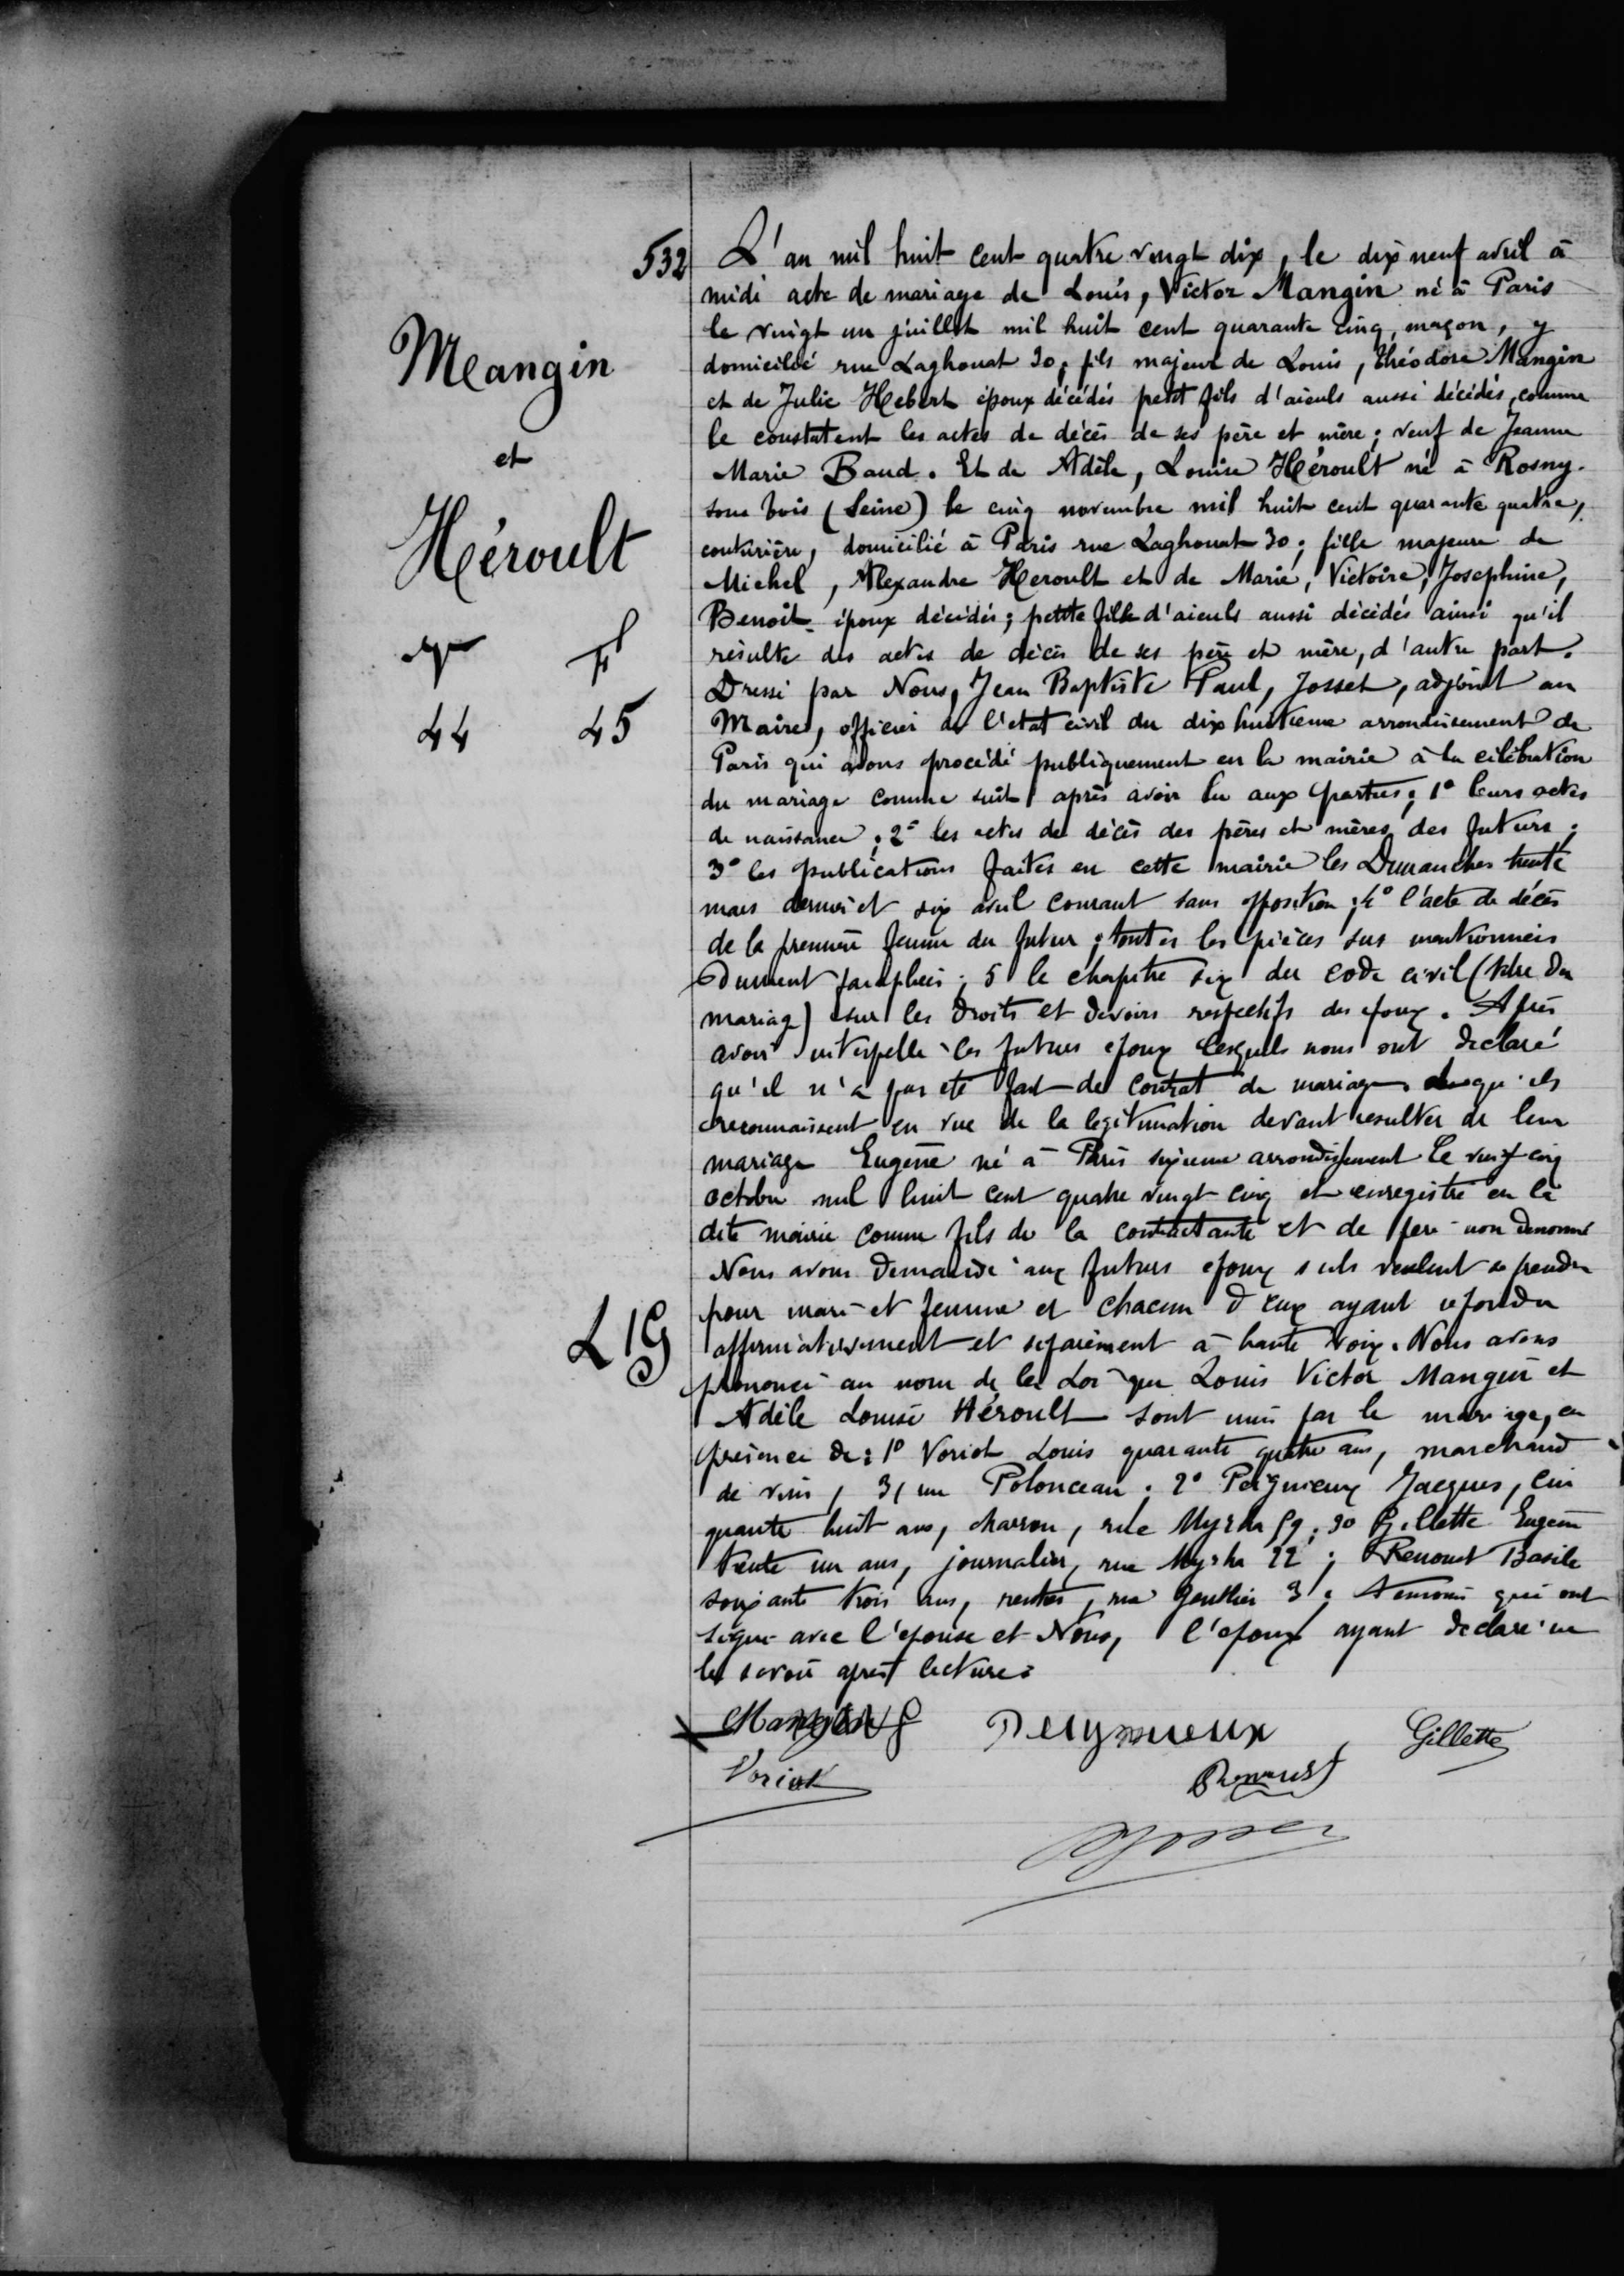532
Meangin
et
Héroult
V F
44 45
L'an mil huit cent quatre vingt dix, le dix neuf avril à
midi acte de mariage de Louis, Victor Mangin né à Paris
le vingt un juillet mil huit cent quarante cinq, maçon, y
domicilié rue Laghoust 90, fils majeur de Louis, Théodore Manfin
et de Julie Hebert époux décédés petit fils d'aieuls aussi décédé comme
le constatent les actes de décès de ses père et mère; veuf de Jeanne
Marie Baud. Et de Adèle, Louise Héroult né à Rosny
sous bois (Seine) le cinq novembre mil huit cent quarante quatre,
couturière, domicilié à Paris rue Laghoust 30. fille majeure de
Michel, Alexandre Heroult et de Marie, Victoire, Josephine,
Benoit époux déédés; petite fille d'aieuls aussi décédés ainsi qu'il
résulte des actes de décès de ses père et mère, d'autre part.
Dressé par Nous, Jean Baptiste Paul, Joseet, adjoint au
Maire, officier de l'etat civil du dix huitième arrondissement de
Paris qui avons procédé publiquement en la mairie à la célébration
du mariage comme suit après vaoir lu aux parties; 1° leurs actes
de naissances, 2° les actes de décès des pères et mères des futurs;
3° les publications faites en cette mairie les Dimanches trente
mars dernier et six avril courant sans opposition; 4° l'acte de décès
de la première femme du futur; toutes les pièces sus mentionnées
dument paraphées; 5 le chapitre six du code civil (titre du
mariage) sur les droits et devoirs respectifs des époux. Après
avoir interpellé les futurs époux lesquels nous ont déclaré
qu'il n'a pas été fait de contrat de mariage puisqu'ils
reconnaissant en vue de la légitimation devant resulter de leur
mariage Eugène né à Paris sixième arrondissement le vingt-cinq
octobre mil huit cent quatre vingt cinq et enregistré en la
dite mairie comme fils de la contractante et de père non denommé
Nous avons demandé aux futurs époux s'ils veulent se prendre
pour mari et femme et chacun d'eux ayant répondu
affirmativement et séparément à haute voix. Nous avons
prononcé au nom de la Loi que Louis Victore Manguin et
Adèle Louise Héroult sont unis par le mariage, en
présence de: 1° Voriot Louis quarante quatre ans, marchand
de vins, 3/, rue Polonceau; 2° Pergnieux Jacques, cin
quante huit ans, Marron, rue Myrha 69; 80 Rillette Eugène
trente un ans, journalier, rue Mysha 22; Renaut Basiile
soixante trois ans, rentier, rue Gauthier 3: témoins qui ont
signé avec l'épouse et Nous, l'époux ayant déclaré ne
le savoir après lecture.
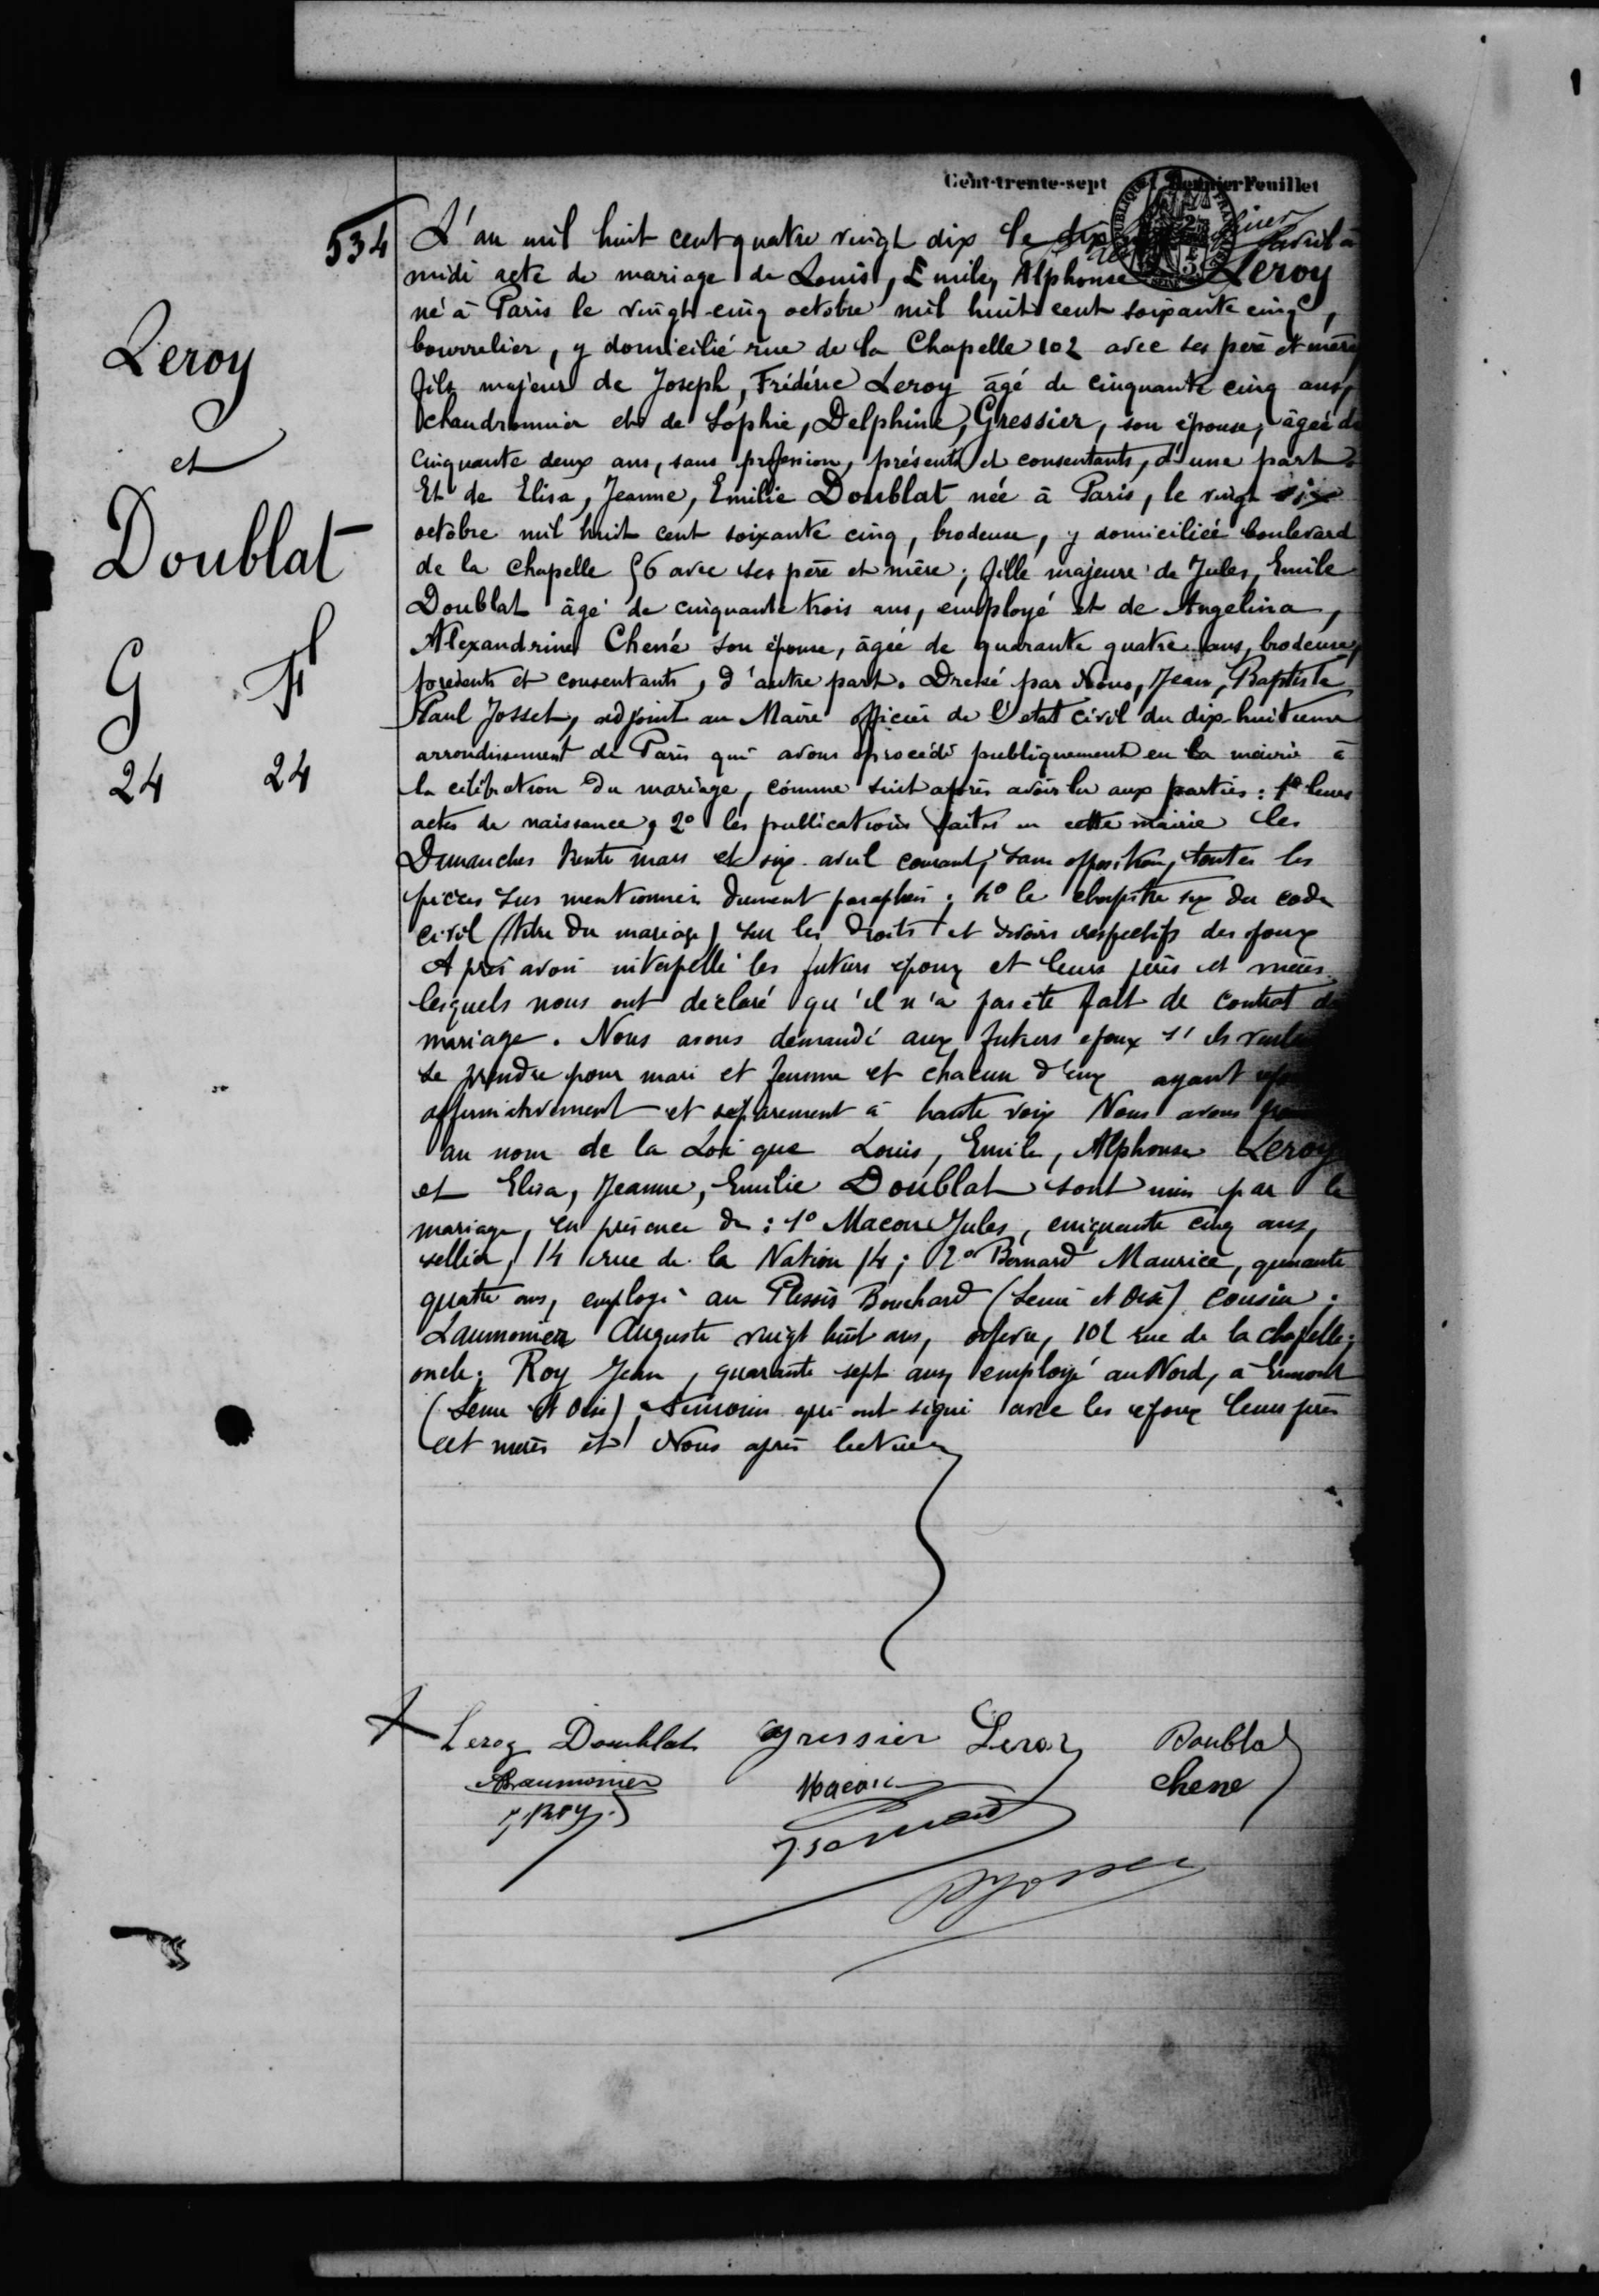543
Leroy
et
Doublat
G F
24 24
L'an mil huit cent quatre vingt dix le dix avril à
midi acte de mariage de Louis, Emile, Alphonse Leroy
né à Paris le vingt cinq octobre mil huit cent soixante cinq
bourrelier, y domicilié rue de la Chapelle 102 avec les père et mère
fils majeur de Joseph, Frédéric Leroy âgé de cinquante cinq ans,
chaudronnier et de Sophie, Delphine, Gressier, son épouse, âgée de
cinquante deux ans, sans profession, présents et consentants, d'une part.
Et de Elisa, Jeanne, Emilie Doublat née à Paris, le vingt six
octobre mil huit cent soixante cinq brodeuse, y domiciliée boulevard
de la Chapelle 56 avec ses père et mère; fille majeure de Jules, Emile
Doublat âgé de cinquante trois ans, employé et de Angelina
Alexandrine Chené son épouse, âgée de quarante quatre ans, brodeuse
présents et consentants, d'autre part. Dressé par Nous, Jean, Baptiste
Paul Josset, adjoint au Maire officier de l'état civil du dix-huitième
arrondissement de Paris qui avons procédé publiquement en la mairie à
la célébration du mariage, comme suit après avoir lu aux parties: 1° leurs
actes de naissance, 2° les publications faites en cette mairie les
Dimanches trente mars et six avril courant sans opposition, toutes les
pièces sus metionnées dument paraphées; 4° le chapitre dix du code
civil (titre du mariage) sur les droits et devoirs respectifs des époux
Après avoir interpellé les futurs époux et leurs pères et mères
lesquels nous ont dclaré qu'il n'a pas été fait de contrat de
mariage.Nous avons demandé aux futurs époux s'ils veul
se prendre pour mari et femme et chacun d'eux ayant répon
affirmativement et séparément à haute voix. Nous avons pro
au nom de la Loi que Louis, Emile, Alphonse Leroy
et Elisa, Jeanne, Emilie Doublat sont unis par le
mariage, en présence de: 1° Macou jules, cinquante cinq ans
sellier, 14 rue de la Nation 14; E° Bernard Maurice, quarante
quatre ans, employé au Plessis Bouchard (Seine et Oiseà cousin
Laumonier Auguste vingt-huit ans, orfevre, 102 rue de la chapelle;
oncle; Roy Jean, quarante sept ans employé au Nord, a Ermont
(Seine et Oise) témoins qui ont signé avec les époux leurs pères
et mères et Nous après lecture